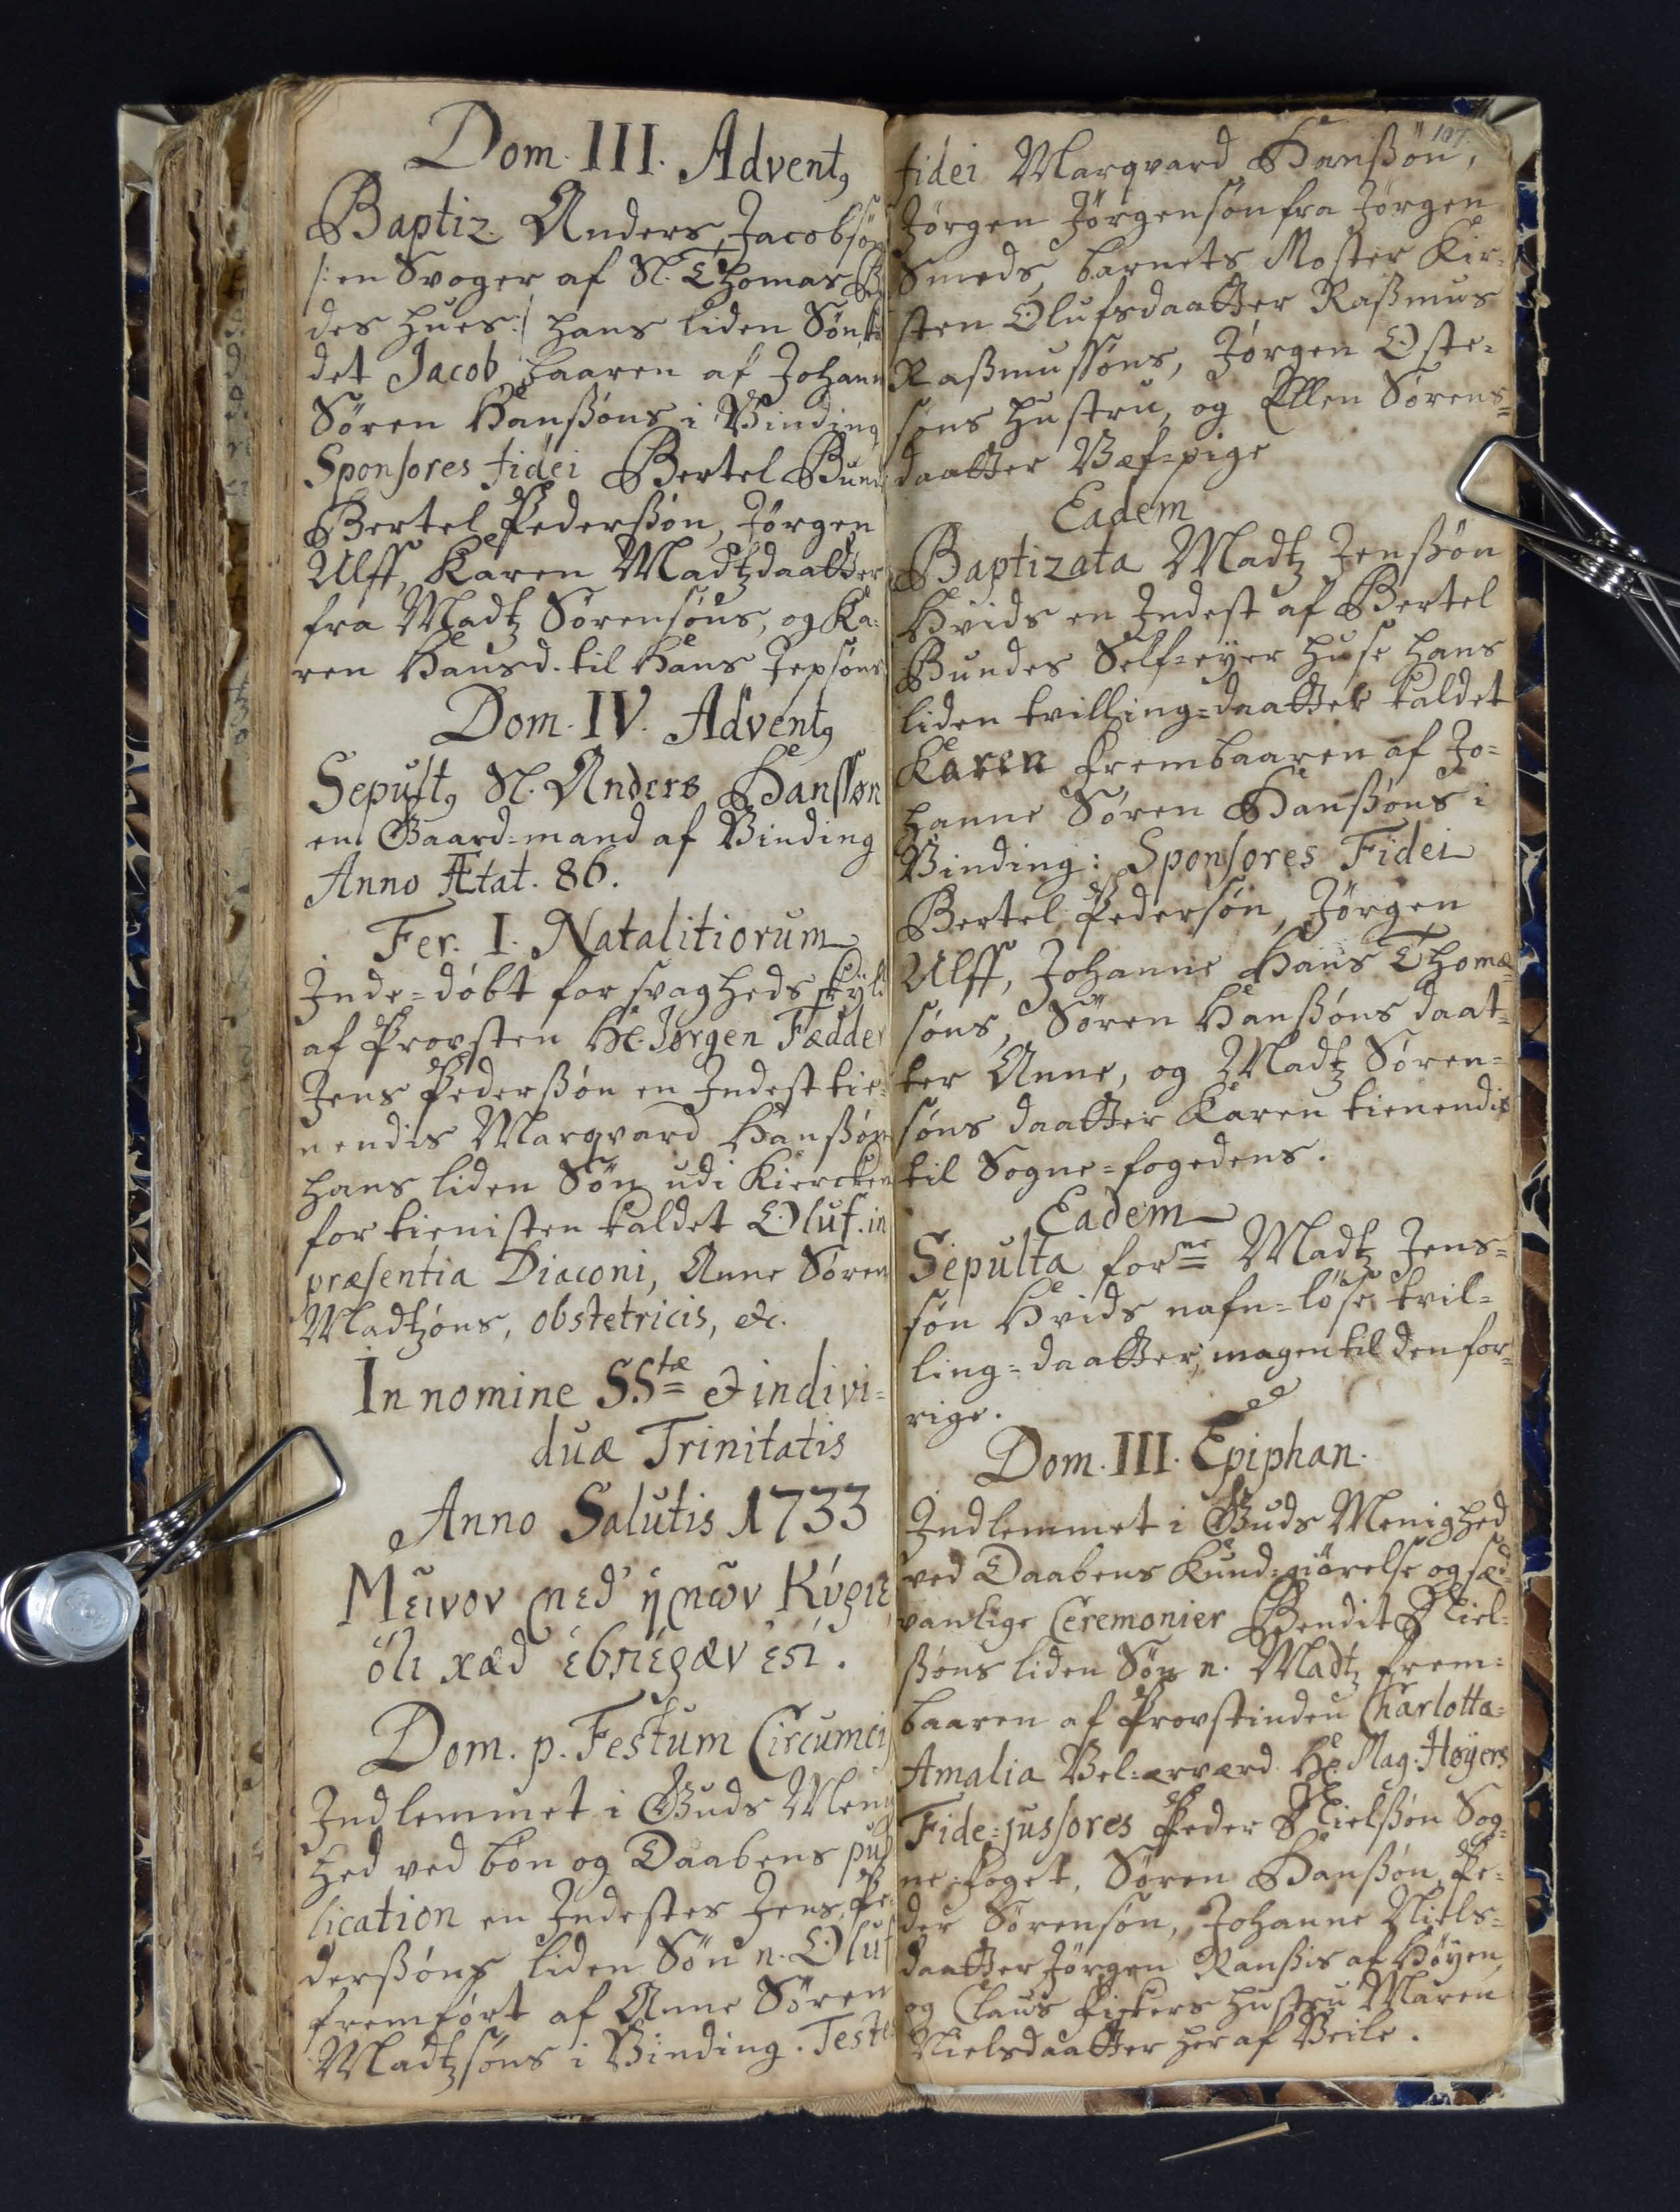Dom. III. Adventq
Baptiz. Anders Jacobsøn
/: en Svoger af Sl. Thomas B  
des hues ./ hans liden Søn ka  
det Jacob, baaren af Johann  
Søren Hanssøns i Vinding
Sponsores fidei Bertel Bund  
Bertel Pederssøn, Jørgen
Ulff, Karen Madtzdaatter
fra Madtz Sørensøns, og Ka¬
ren Hansd. til Hans Jepsøns
Dom. IV. Adventq
Sepultq Sl. Anders Hanssøn
en Gaard=mand af Vinding
Anno Ætat. 86.
Fer. I. Natalitiorum
Inde=døbt for svagheds skyld
af Præsten Hl Jørgen Fædder
Jens Pederssøn en Indest tie¬
nendis Marqvard Hanøn,
hans liden Søn udi Kiercken
for tienisten kaldet Oluf. in
præsentia Diaconi, Anne Søren 
Madtzøns, obstetricis, etc.
In nomine SStæ et indivi¬
duæ Trinitatis
Anno Salutis 1733
Meivov ned ## ## Kvqie
 oli xæd eb## ##.
Dom. p. Festum Circumci  
Indlemmet i Guds Menig
hed ved bøn og Daabens pub  
lication en Indestes Jens Pe
derssøns liden Søn n. Oluf  
fremført af Anne Søren
Madtzsøns i Vinding. Testes  
107
fidei Marqvard Hanssøn,
Jørgen Jørgensøn fra Jørgen
Smeds, barnets Moster Kir¬
sten Olufsdaatter Rassmus
Rassmussøns, Jørgen Oste¬
søns hustru, og Ellen Sørens¬
daatter Væf=pige
Eadem
Baptizata Madtz Jenssøn
Hvids en Indest af Bertel
Bundes Self=eyer huse hans
liden tvilling=daatter kaldet
Karen, frembaaren af Jo¬
hanne Søren Hanssøns i
Vinding: Sponsores Fidei
Bertel Pedersøn, Jørgen
Ulff, Johanne Hans Thomæ¬
søns, Søren Hanssøns daat¬
ter Anne, og Madtz Søren¬
søns daatter Karen tienendis
til Sogne=fogedens.
Eadem
Sepulta forne Madtz Jens¬
søn Hvids nafn=løse tvil¬
ling=daatter magen til den for¬
rige.
Dom. III. Epiphan.
Indlemmet i Guds Menighed
ved Daabens Kund=giørelse og sæd¬
vanlige Ceremonier Bendit Niel¬
ssøns liden Søn n. Madtz, frem¬
baaren af Provstinden Charlotta =
Amalia Vel=ærværd. Hl. Mag. Høyers
Fide=jussores Peder Nielssøn Sog¬
ne=Foget, Søren Hanssøn, Pe¬
der Sørensøn, Johanne Niels¬
daatter Jørgen Ranssis af Høyen,
og Claus Fiskers hustru Maren
Nielsdaatter her af Veile.
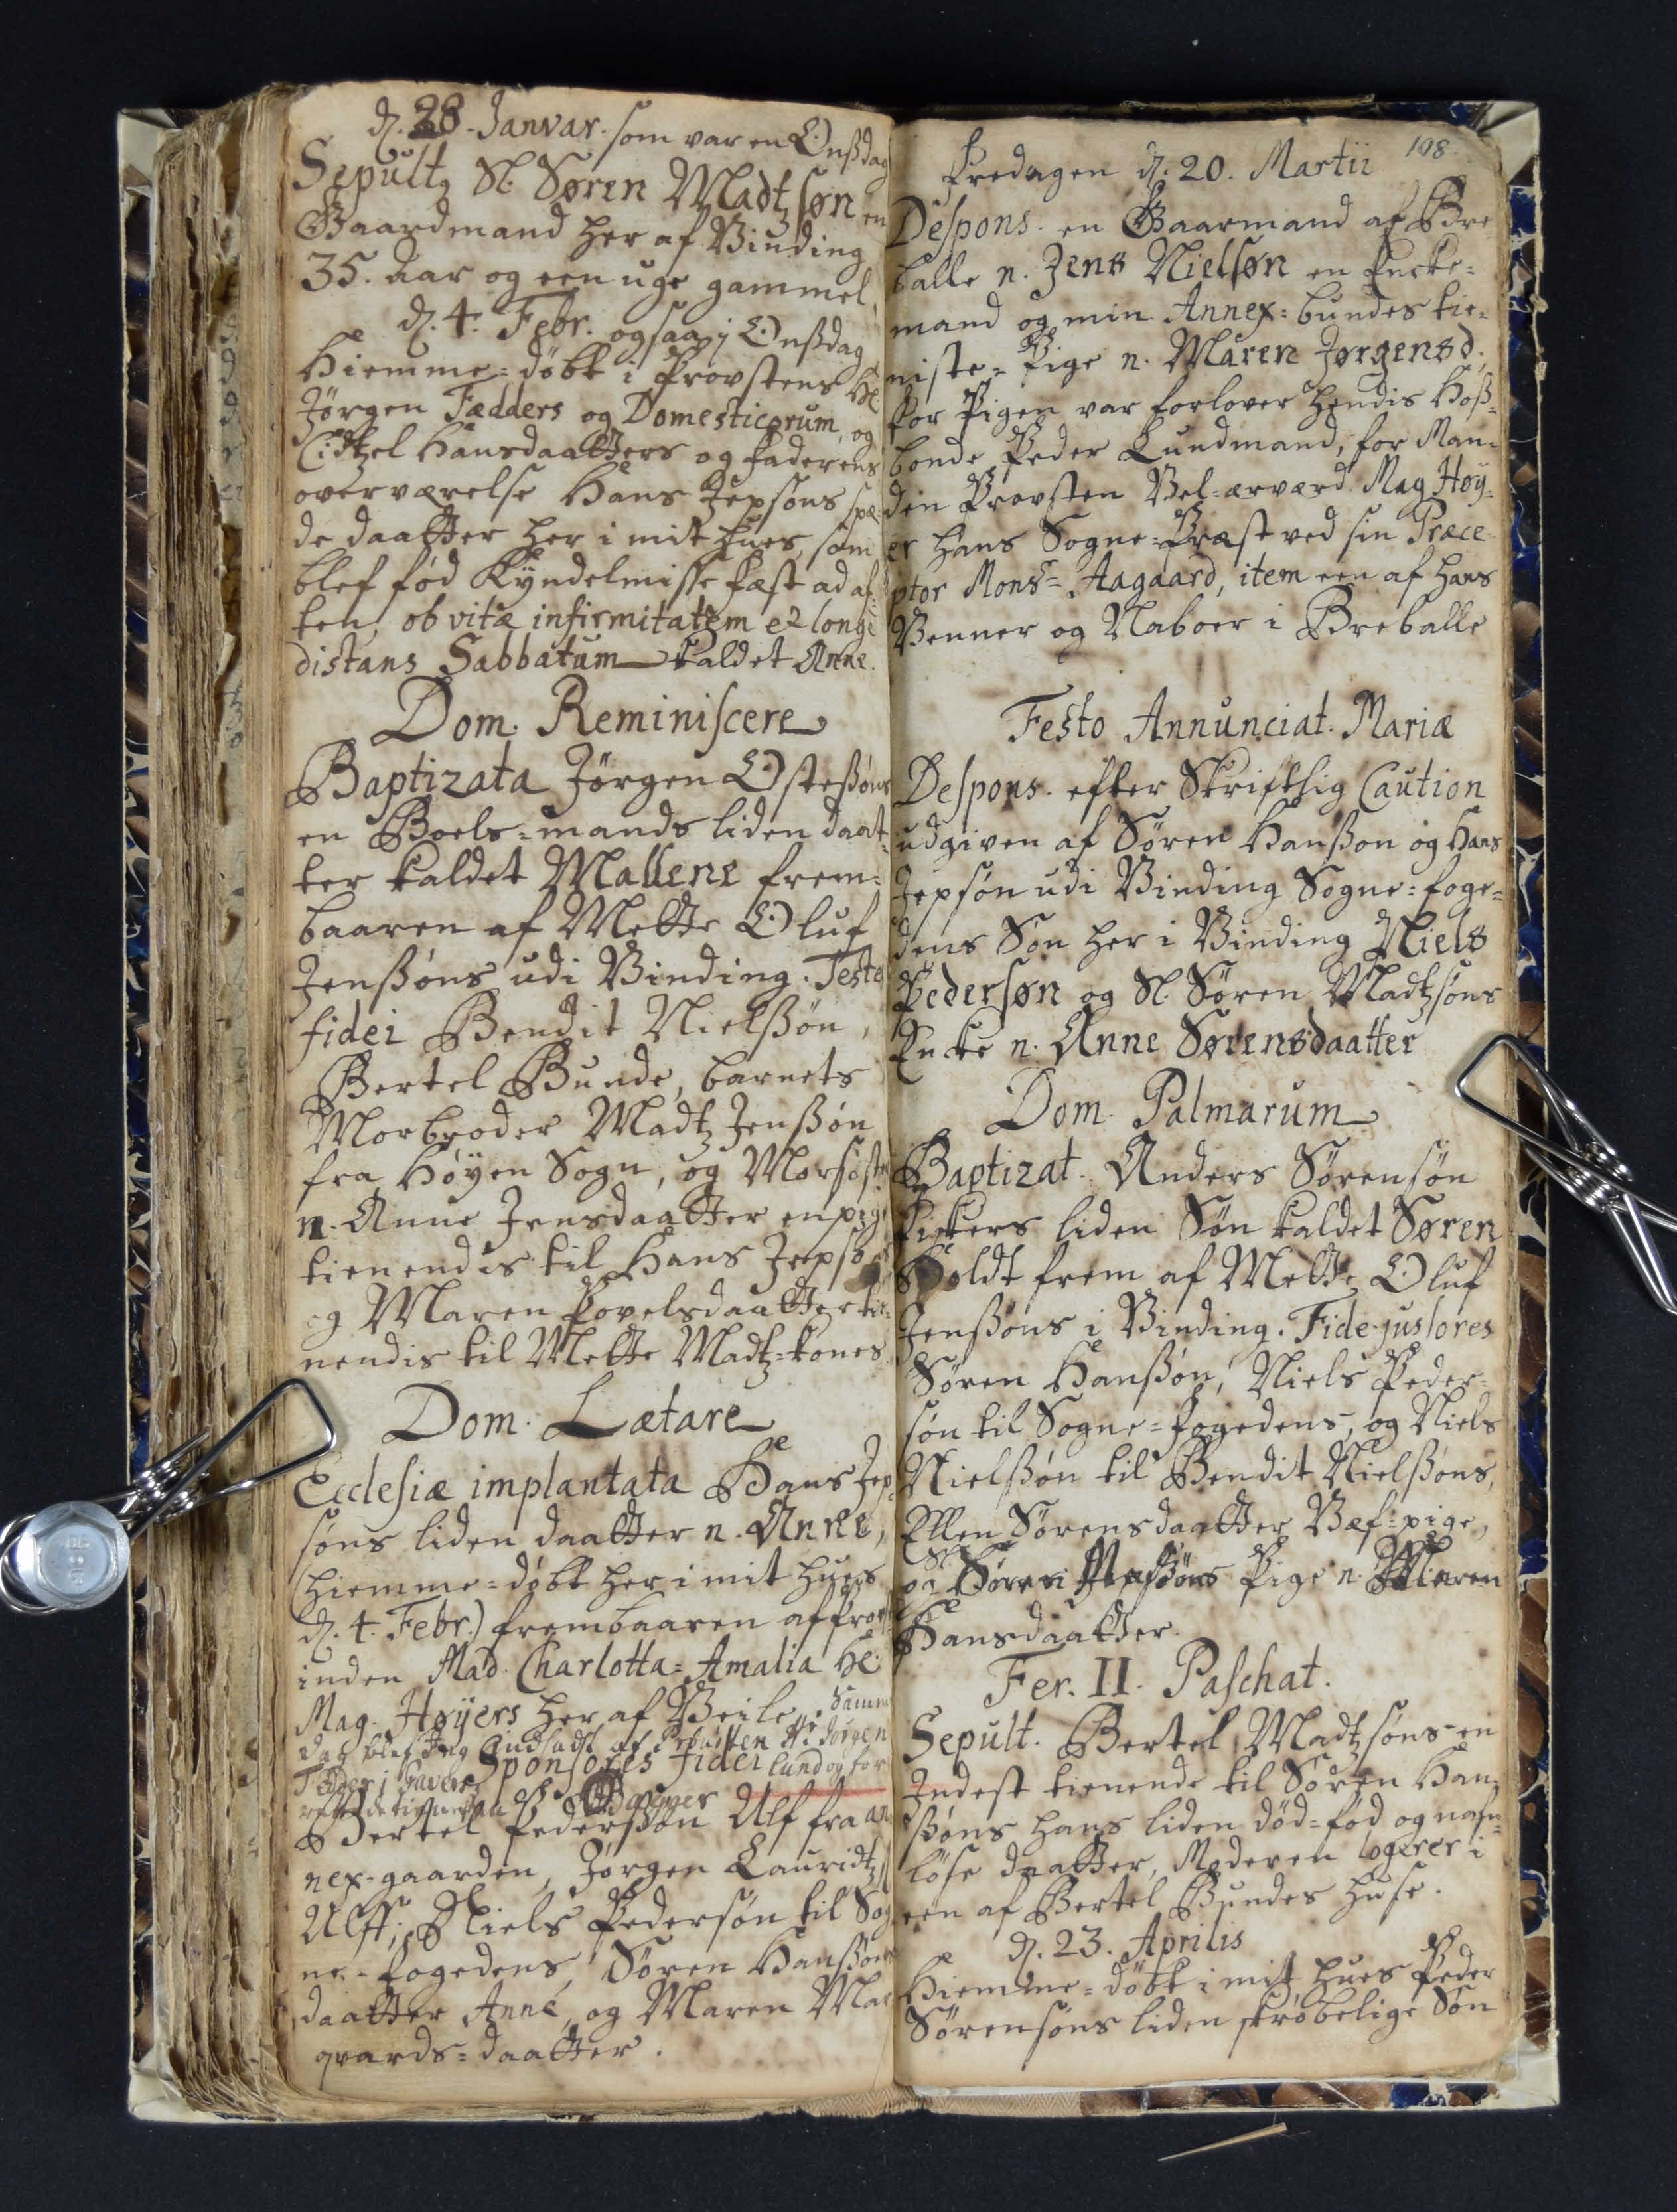d. 28. Janvar. som var en Onssdag
Sepultq Sl. Søren Madtzsøn en
Gaardmand her af Vinding 
35. Aar og een uge gammel
d. 4. Febr. ogsaa 1 Onsdag
hiemme=døbt i Provstens Hl.
Jørgen Fædders og Domesticorum, og
Cidtzel Hansdaatters og Faderens
overværelse Hans Jepsøns spæ¬
de daatter her i mit hues, som
blef fød Kyndelmisse Fæst ad af¬
ten, ob vitæ infirmitatim et longe
distans Sabbatum kaldet Anne.
Dom. Reminiscere
Baptizata Jørgen Ostessøns
en Boels=mands liden daat¬
ter kaldet Mallene frem¬
baaren af Mette Oluf
Jenssøns udi Vinding. Testes
fidei Bendit Nielssøn
Bertel Bunde, barnets
Morbroder Madtz Jenssøn
fra Høyen Sogn, og Morsøster  
n. Anna Jensdaatter en pige
tienendis til Hans Jep 
og Maren Povelsdaatter tie  
nendis til Mette Madtz=Kones
Dom. Lætare
Ecclesiæ implantata Hans Jep¬
søns liden daatter n. Anne,
(hiemme=døbt her i mit hues
d. 4. Febr.) frembaaren af Provst¬  
inden Mad. Charlotta= Amalia Hl.
Mag. Høyers her af Veile. Samm
dag blef Jeg indsadt af Prousten H## Jørgen
Fædder## i Gavers
lund og for
rettede tienesten O Bagger
Sponsores fidei
Bertel Pederssøn Ulf fra an
nex-gaarden, Jørgen Lauridtz
Ulff; Niels Pederssøn til Sog¬
ne=Fogedens, Søren Hanssøns
daatter Anne, og Maren Mar  
qvards=daatter
108.
Fredagen d. 20. Martii
Despons. en Gaardmand af Bre¬
balle n. Jens Nielsøn en Encke¬
mand og min Annex=bundes tie¬
niste = Pige n. Maren Jørgensd.
For Pigen var forlover hendis Hoss¬
bonde Peder Lundmand, for Man¬
den Provsten Vel=ærværd. Mag Høy¬
er hans Sogne=ræst ved sin Præce¬
ptor Monsr Aaagaard, item een af hans
Venner og Naboer i Breballe
Festo Annunciat. Mariæ
Despons. efter Skriftlig Caution
udgiven af Søren Hanssøn og Hans
Jepsøn udi Vinding, Sogne=Foge¬
dens Søn her i Vinding Niels
Pedersøn og Sl. Søren Madtzsøns
Encke n. Anne Sørensdaatter
Dom. Palmarum
Baptizat. Anders Sørensøn
Fiskers liden Søn kaldet Søren
holdt frem af Mette Oluf
Jenssøns i Vinding. Fide-jusores
Søren Hanssøn, Niels Peder¬
søn til Sogne=Fogedens, og Niels
Nielssøn til Bendit Nielssøns,
Ellen Sørensdaatter Væf=pige,
Sl.
og Søren Madsøns Pige n. Maren
Hansdaatter.
Fer. II. Paschat.
Sepult. Bertel Madtzsøns en
Indest tienende til Søren Han¬
ssøns hans liden død=fød og nafn¬
løse daatter, Moderen logerer i
een af Bertel Bundes huse
d. 23. Aprilis
Hiemme=døbt i mit hues Peder
Sørensøns liden skrøbelige Søn